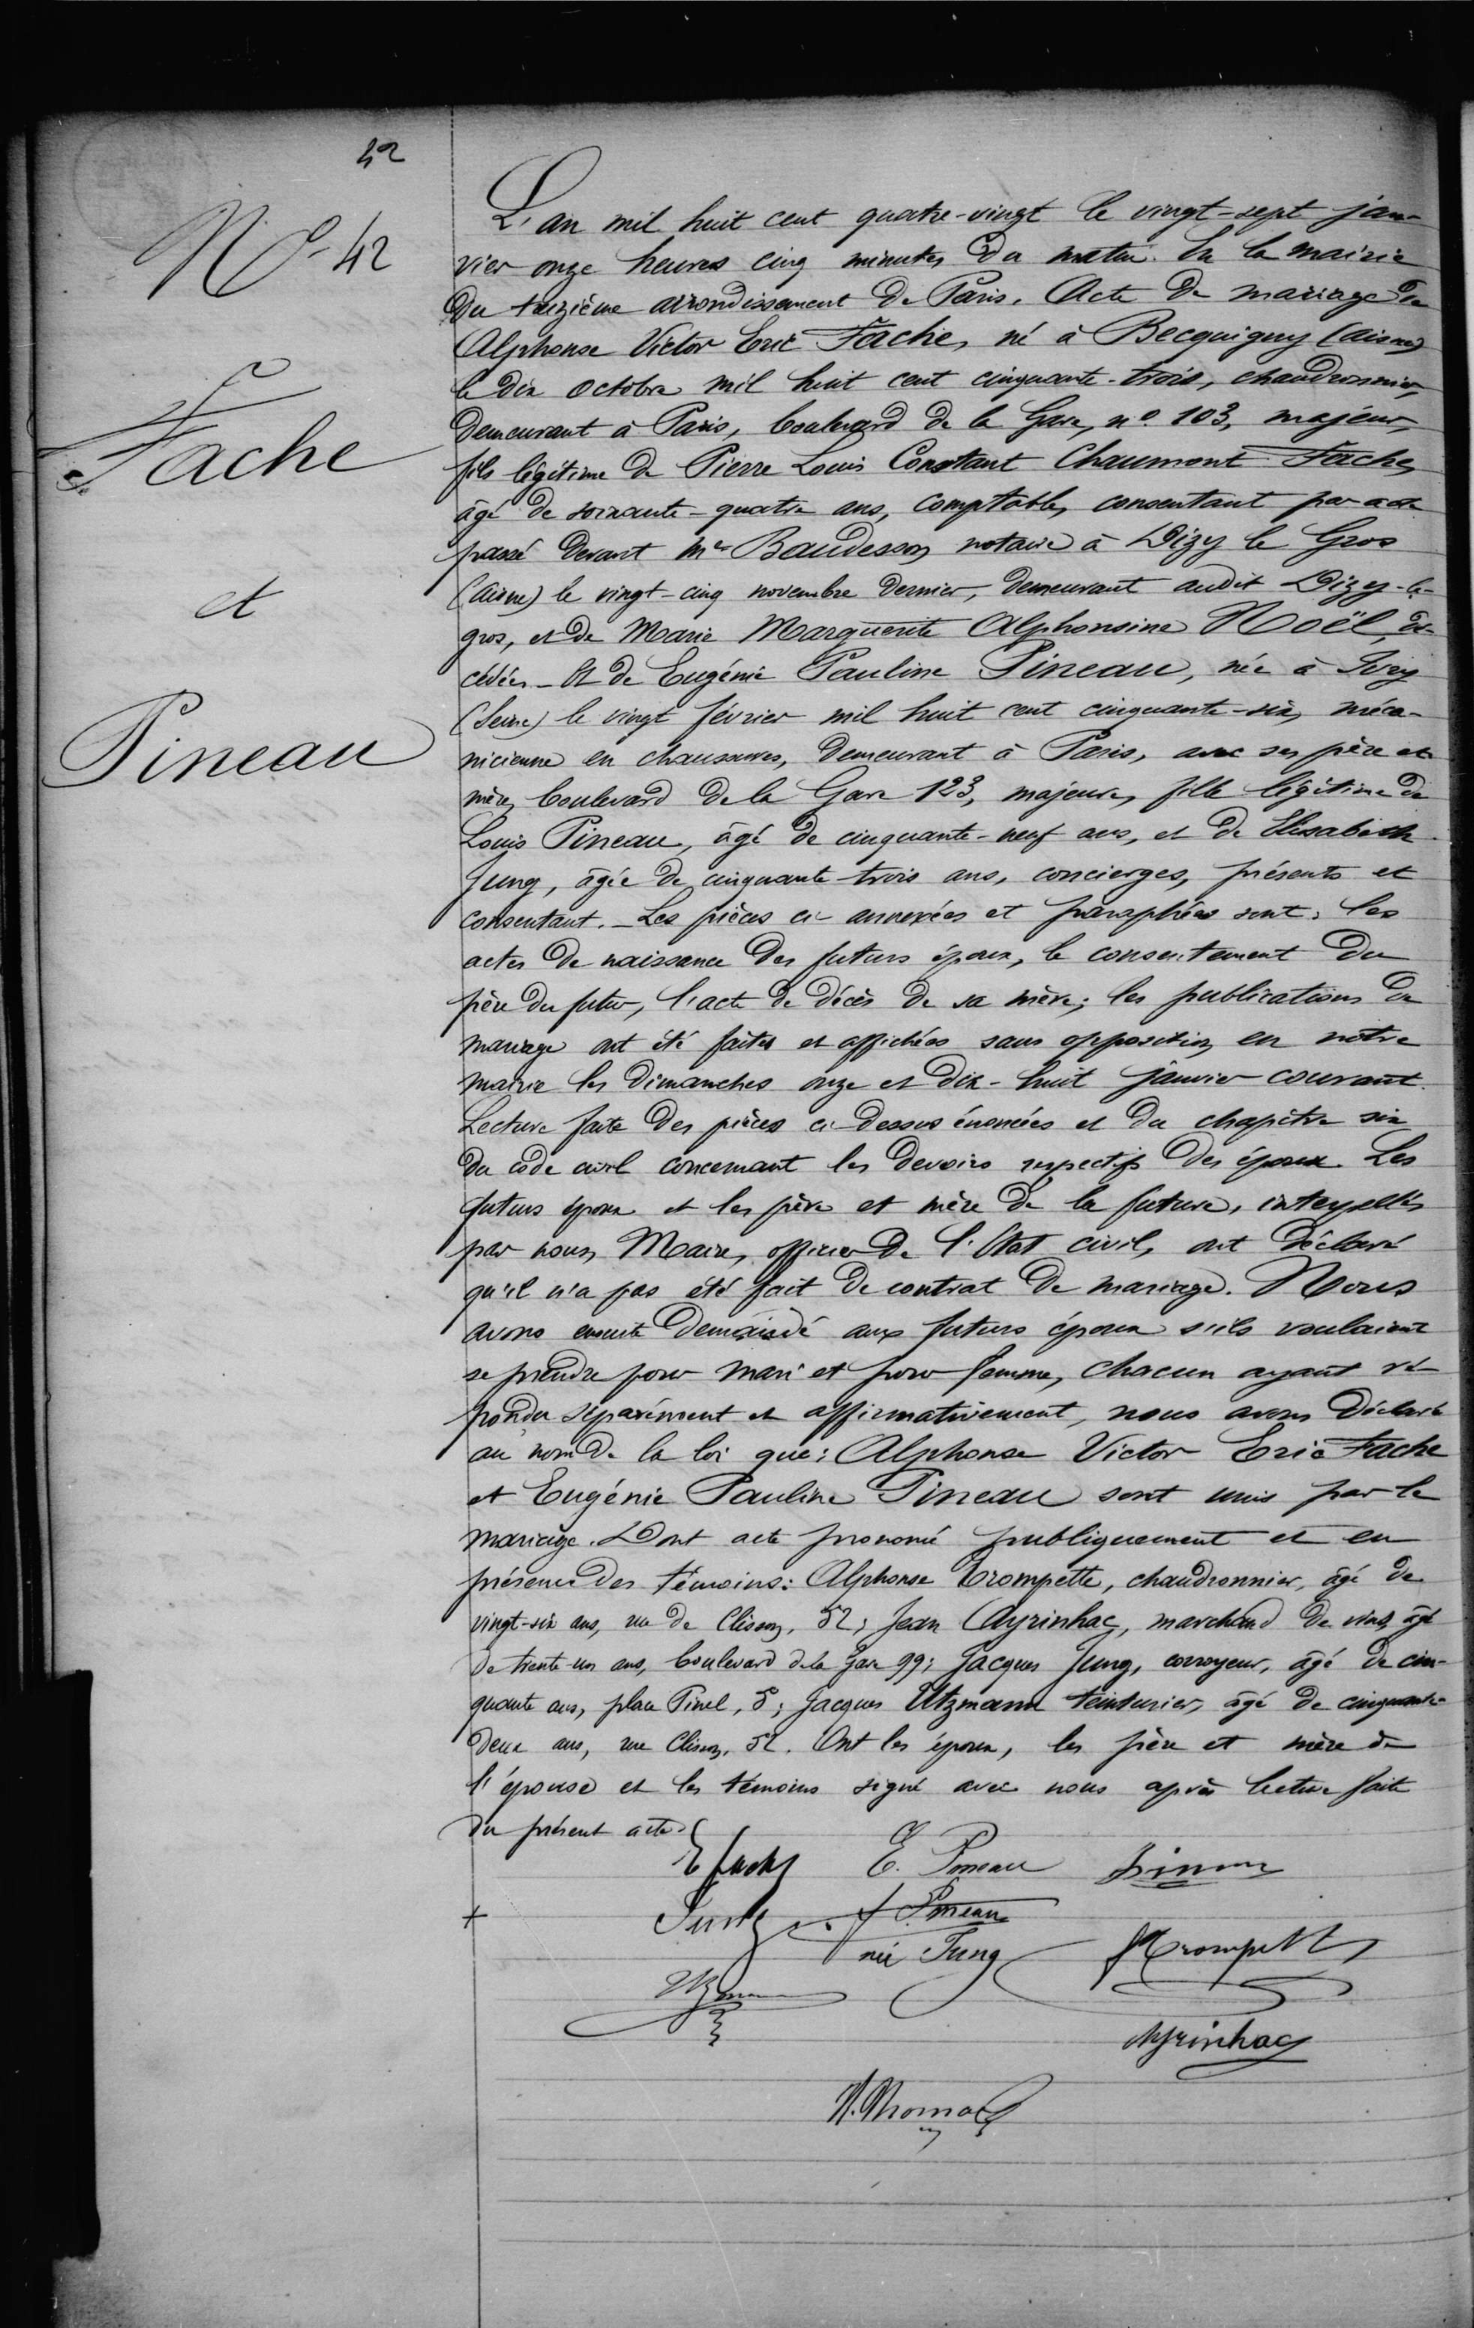N°42
Fache
et
Pineau
L'an mil huit cent quatre vingt le vingt-sept jan-
vier onze heures cinq minutes du matin. En la mairie
du treizième arrondissement de Paris, Acte de mariage de
Alphonse Victor Eric Fache, né à Becquigney (Aisne)
le dix octobre mil huit cent cinquante-trois, chaudronnier,
demeurant à Paris, boulevard de la Gare, n°103, majeur,
fils légitime de Pierre Louis Constant Chaumont Fache,
âgé de soixante-quatre ans, comptable, consentant par acte
passé devant M° Baudesson notaire à Dizy le Gros
(Aisne) le vingt-cinq novembre dernier, demeurant audit Dizy-le-
Gros, et de Marie Marguerite Alphonsine Noël, dé
cédées Et de Eugénie Pauline Pinceau, née à Puey
(Seine) le vingt février mil huit cent cinquante-six, méca-
nicienne en chaussures, demeurant à Paris, avec ses père et
mère, boulevard de la Gare 123, majeure, fille légitime de
Louis Pineau, âgé de cinquante-neuf ans, et de Elisabeth
Jung, âgée de cinquante trois ans, concierges, présents et
consentant. Les pièces ci annexées et paraphées sont les
actes de naissance des futurs époux, le consentement du
père du futur, l'acte de décès de sa mère; les publications de
mariage ont été faites et affichées sans opposition, en notre
mairie les dimanches onze et dix-huit janvier courant.
Lecture faite des pièces ci-dessus énoncées et du chapitre six
du code civil concernant les devoirs respectifs des époux. Les
futurs époux et les père et mère de la future, interpellés
par nous Maire, officier de l'Etat civil, ont déclaré
qu'il n'a pas été fait de contrat de mariage. Nous
avons ensuite demandé aux futurs époux s'ils voulaient
se prendre pour mari et pour femme, chacun ayant ré
pondu séparément et affirmativement, nous avons déclaré
au nom de la loi que: Alphonse Victor Eric Fache
et Eugénie Pauline Pineau sont unis par le
mariage. dont acte prononcé publiquement et en
présence des témoins: Alphonse Trompette, chaudronnier, âgé de
vingt-six ans, rue de Clissy, 52, Jean Layrinhac, marchand de vins, âgé
de trente un ans, boulevard de la Gare 99; Jacques Jung, covoyeur, âgé de cin-
quante ans, place Pinel, 3, Jacques Utzmann teinturier, âgé de cinquante-
deux ans, rue Clissy, 52. Ont les époux, les père et mère de
l'épouse et les témoins signé avec nous après lecture faite
du présent acte
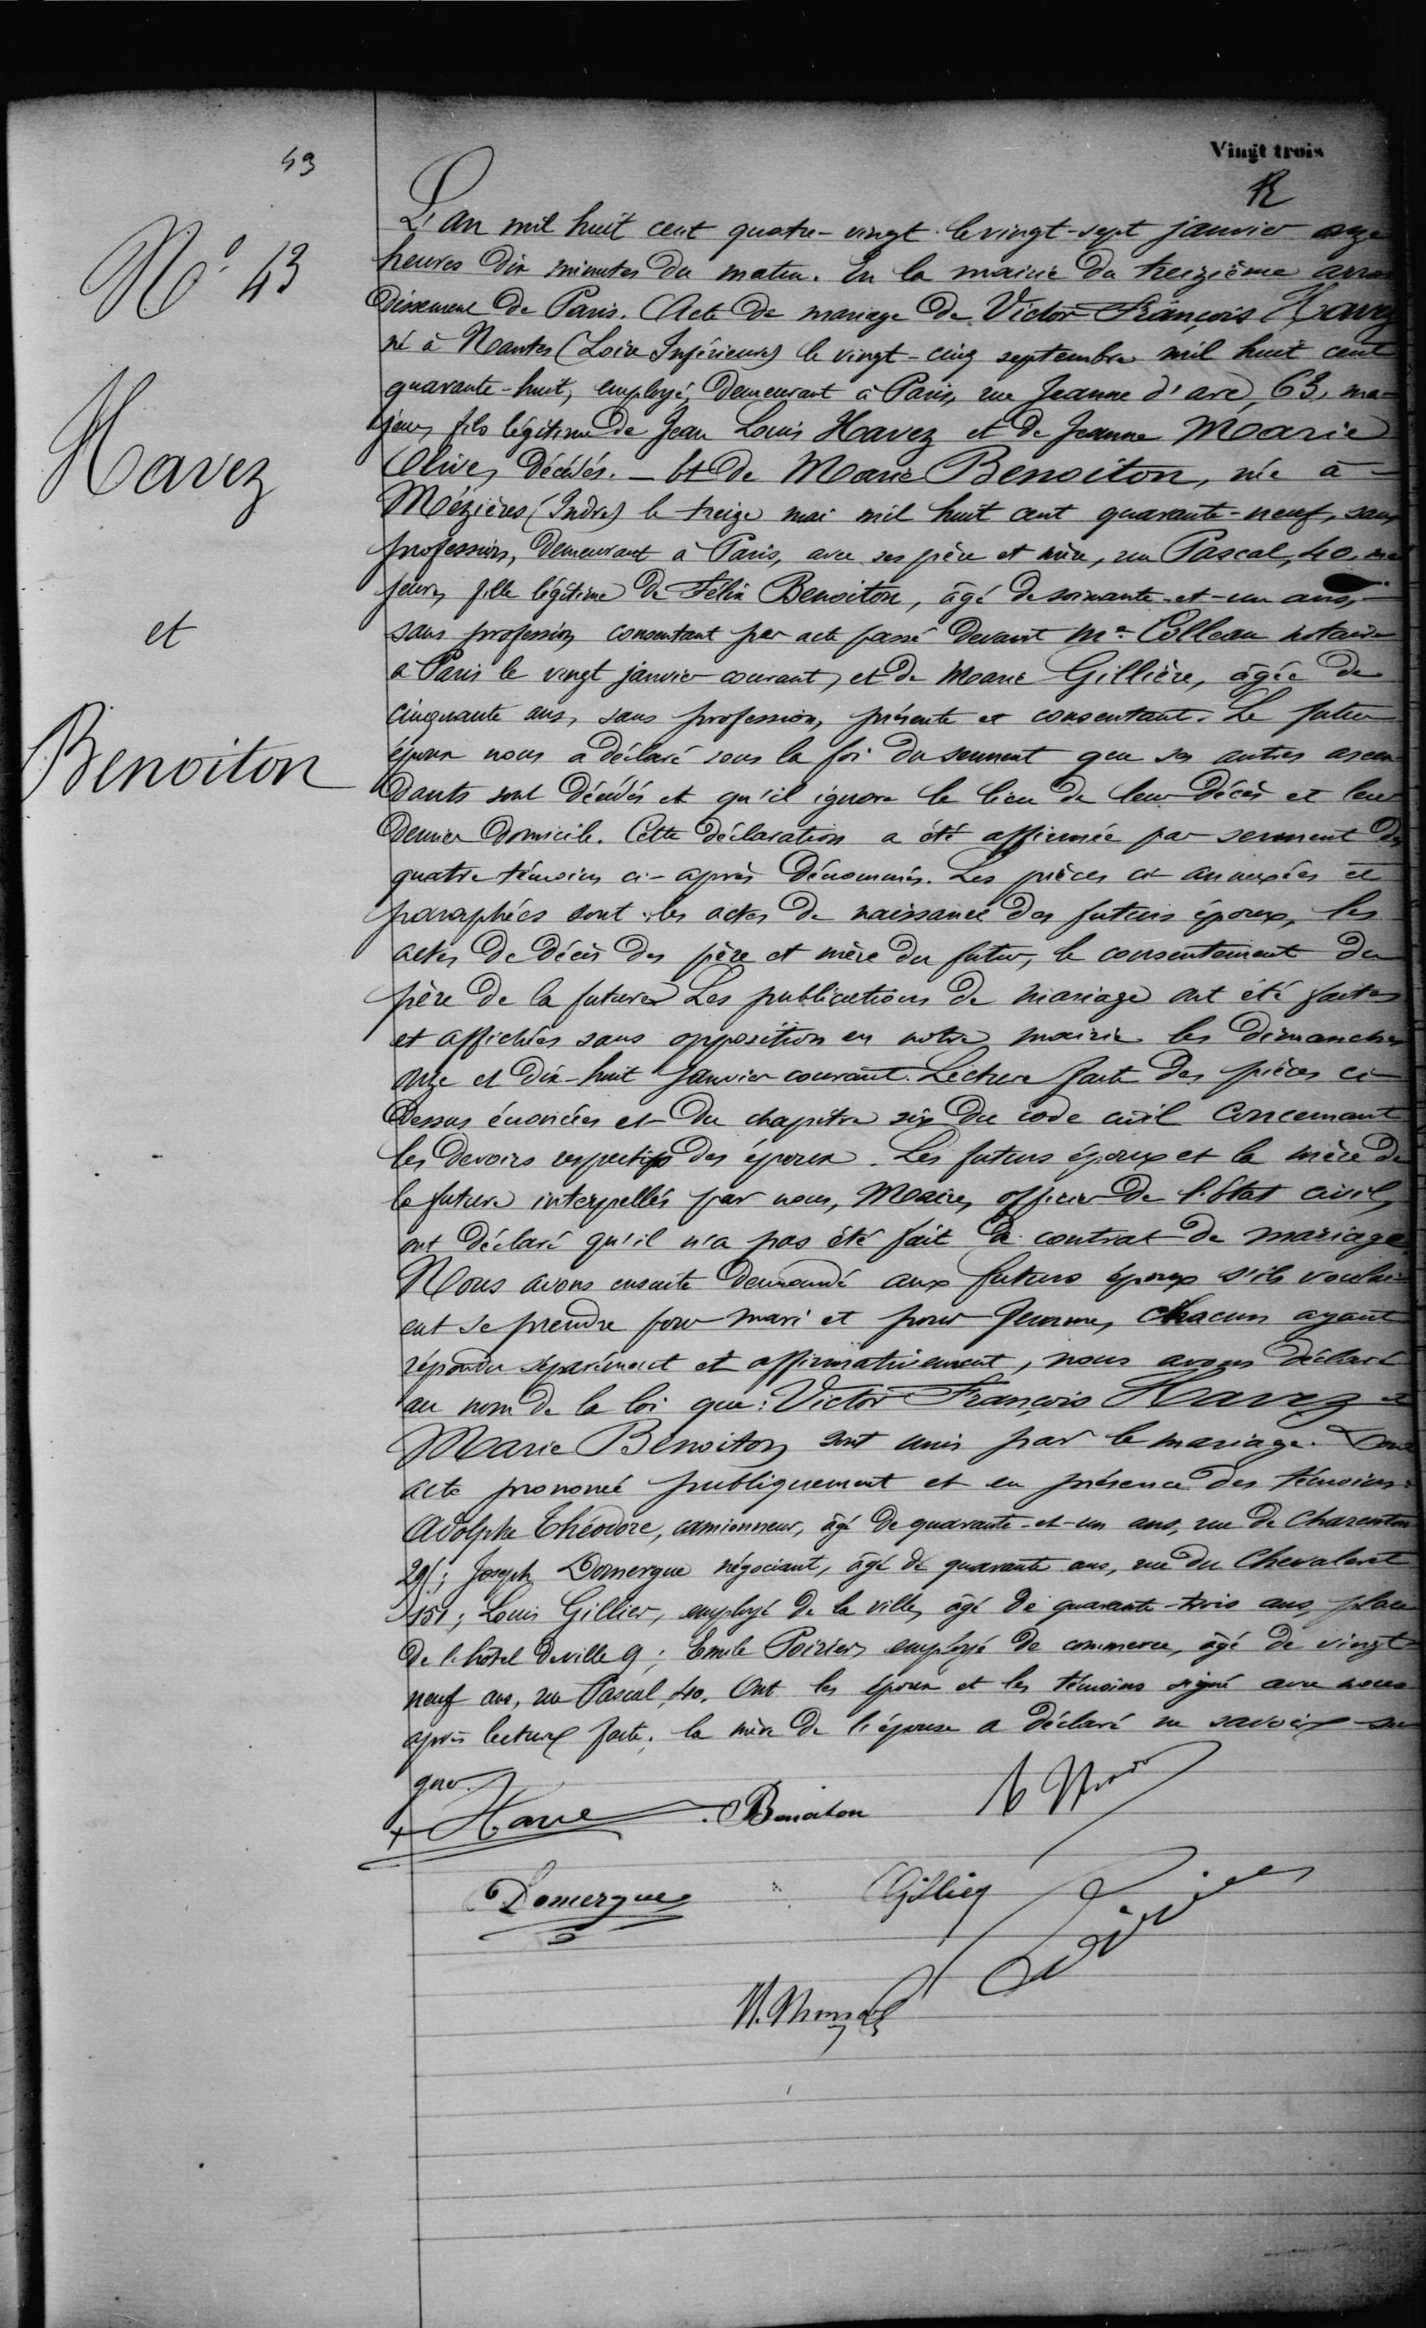N°43
Havez
et
Benoiton
L'an mil huit cent quatre-vingt, le vingt-sept janvier onze
heures dix minutes du matin. En la mairie du treizième arron
dissement de Paris. Acte de mariage de Victor François, Havez
né à Nantes (Loir Inférieur) le vingt-cinq septembre mil huit cent
quarante-huit, employé demeurant à Paris rue Jeanne d'arc, 63, ma
jeur, fils légitime de Jean Louis Havey et de Jeanne Marie
Olive, décédés.-Et de Marie Benoiton, née à
Mézières (Indre) le treize mai mil huit cent quarante-neuf sans
profession, demeurant à Paris, avec ses père et mère, rue Pascal, 40, ma
jeur, fille légitime de Félix Benoiton, âgé de soixante-et-un ans,
sans profession, consentant par acte passé devant M° Colleau, notaire
à Paris le vingt janvier courant, et de Marie Gillière, âgée de
cinquante ans, sans profession, présente et consentant. Le futur
époux nous a déclaré sous la foi du serment que ses autres ascen
dants sont décédés et qu'il ignore le lieu de leur décès et leur
dernier domicile. Cette déclaration a été affirmée par serment des
quatre témoins ci-après dénommés. Les pièces ci annexées et
paraphées sont: les actes de naissance des futurs époux, les
actes de décès des père et mère du futur, le consentement du
père de la future. Les publications de mariage ont été faits
et affichées sans opposition en notre mairie les dimanches
onze et dix-huit janvier courant. Lecture faite des pièces ci-
dessus énoncées et du chapitre six du code civil concernant
les devoirs respectifs des époux. Les futurs époux et la mère de
la future interpellés par nous, Maire, officier de l'Etat civil,
ont déclaré qu'il n'a pas été fait de contrat de mariage.
Nous avons ensuite demandé aux futurs époux s'ils voulai
ent se prendre pour mari et pour femme, chacun ayant
répondu séparément et affirmativement, nous avons déclaré
au nom de la loi que: Victor François Havez &
Marie Benoiton sont unis par le mariage. Dont
acte prononcé publiquement et en présence des témoins
Adolphe Théodore, camionneur, âgé de quarante-et-un ans, rue de Charenton
291; Joseph Domergue négociant, âgé de quarante ans, rue du Chevaleret
151; Louis Gillier, employé de la ville, âgé de quarante-trois ans, place
de l'hôtel de ville 9: Emile Poirier employé de commerce, âgé de vingt
neuf ans, rue Pascal, 40, Ont les époux et les témoins signé avec nous
après lecture faite, la mère de l'épouse a déclaré ne savoir si
gner.